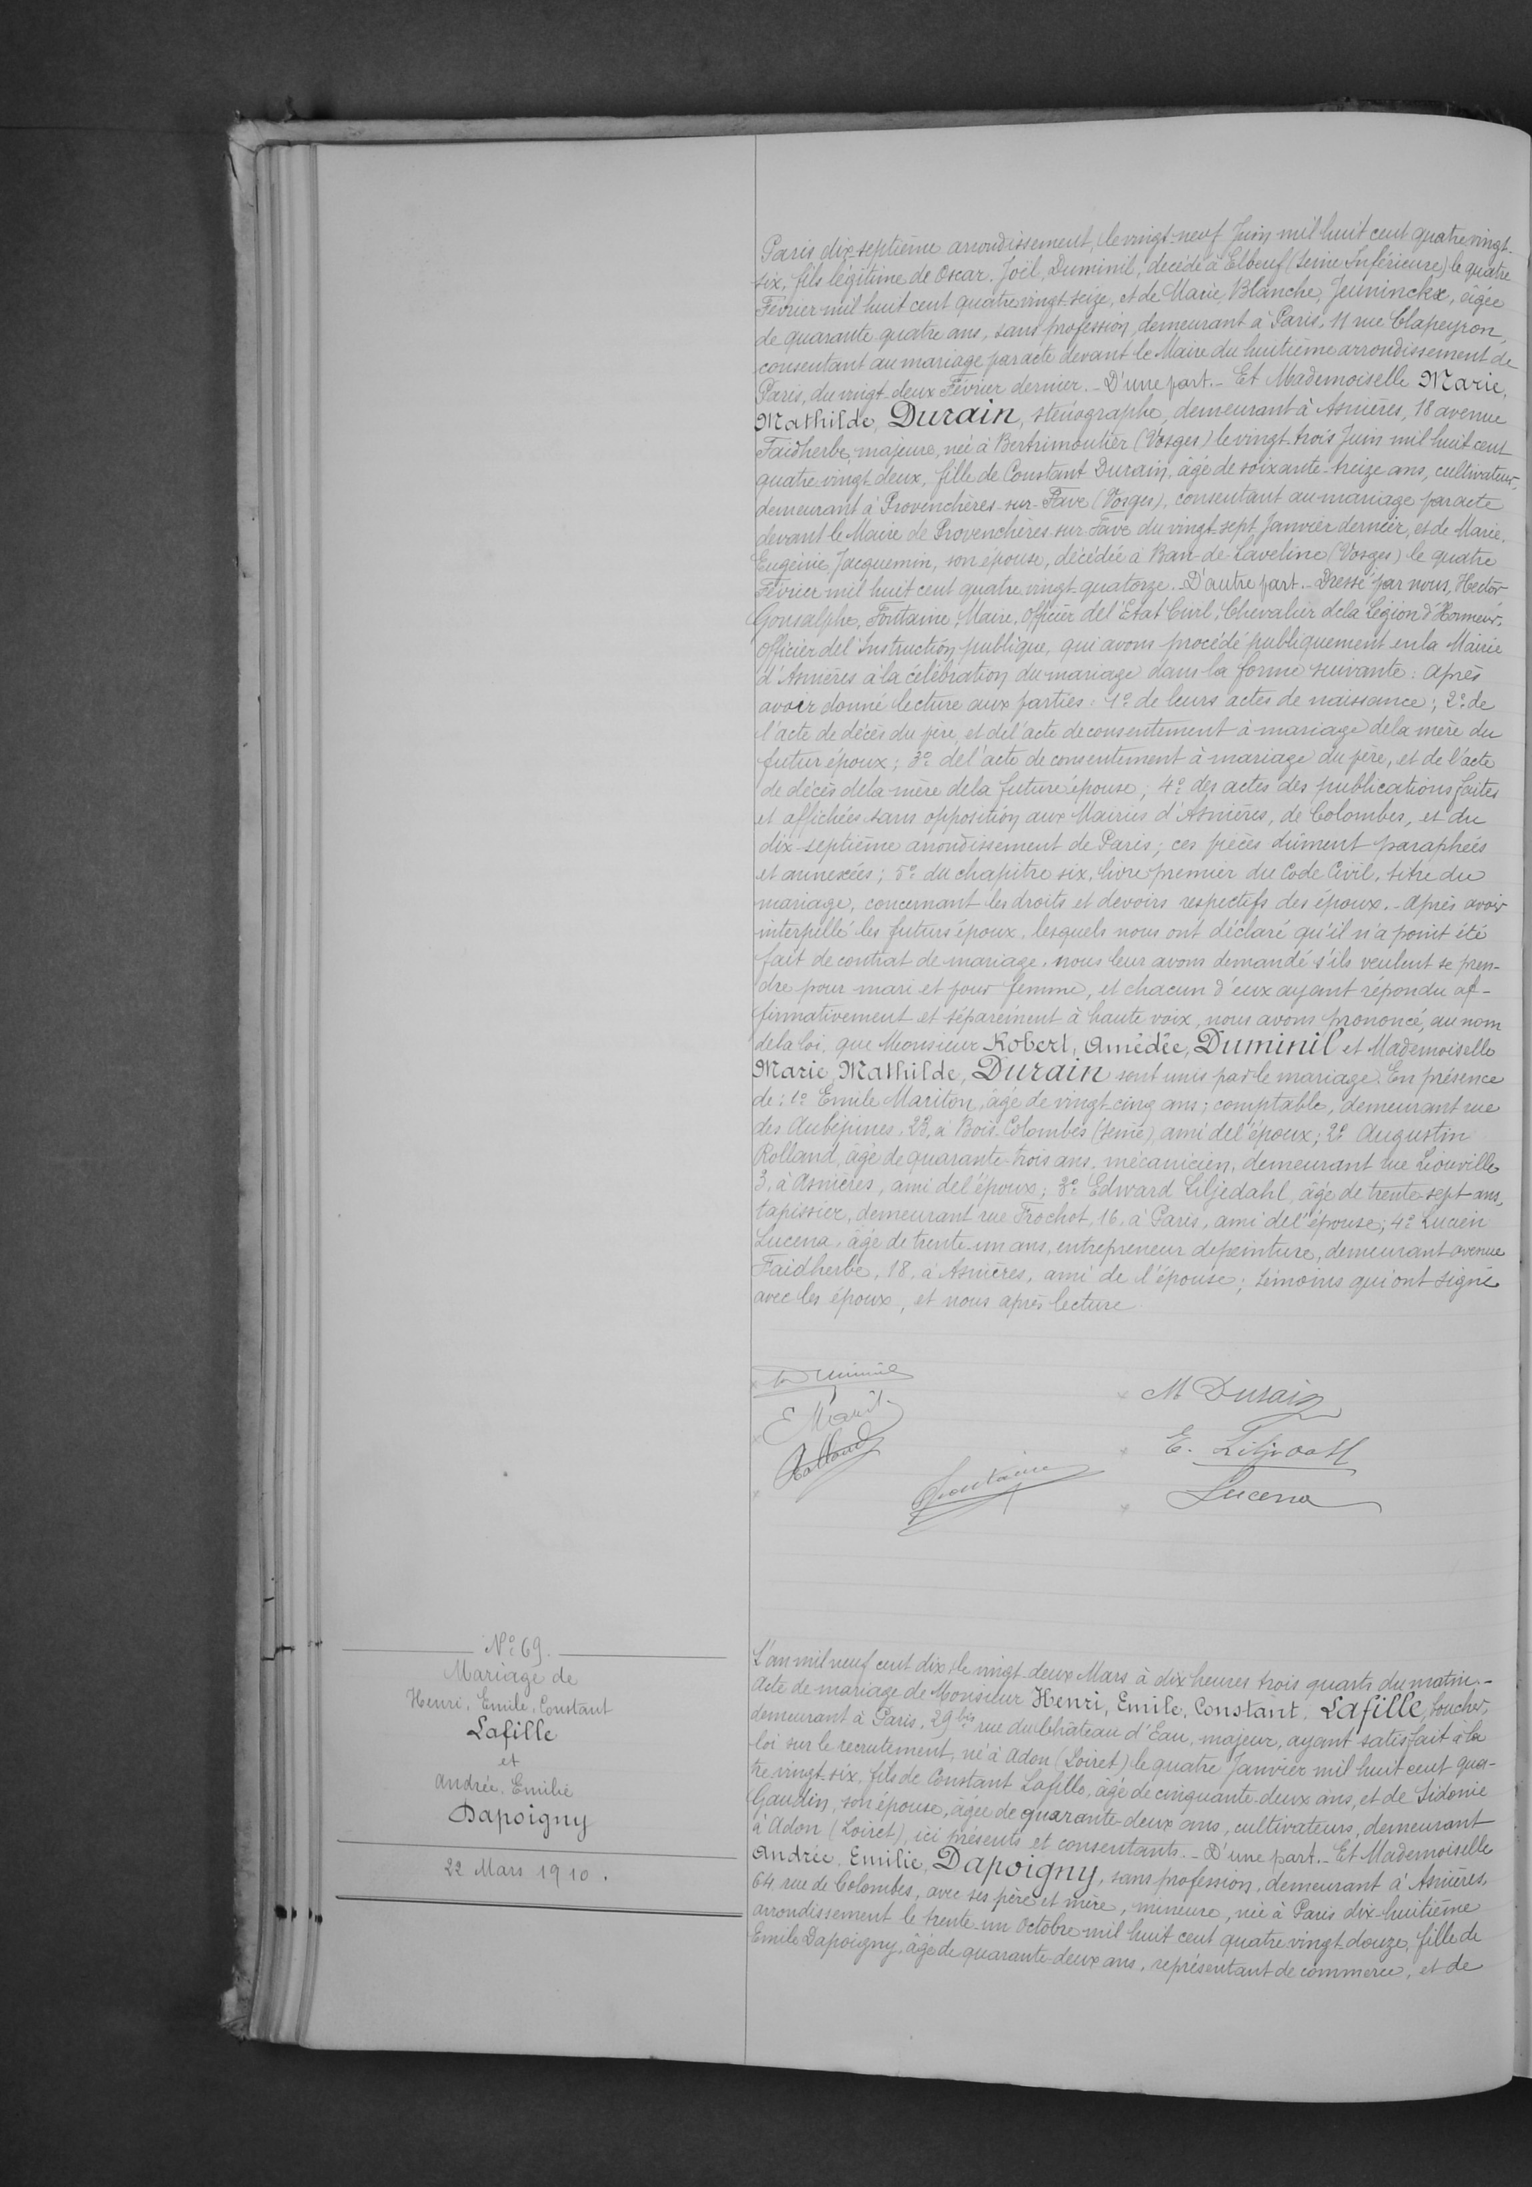Paris dix septième arrondissement, le vingt neuf Juin mil huit cent quatre vingt
six, fils légitime de Oscar. Joël Duminil, décédé à Elbeuf (Seine Inférieure) le quatre
Février mil huit cent quatre vingt seize et de Marie Blanche Jeuninckx, âgée
de quarante quatre ans, sans profession demeurant à Paris, 11 rue Clapeyron,
consentant au mariage par acte devant le Maire du huitième arrondissement de
Paris, du vingt-deux Février dernier.-D'une part .-Et Mademoiselle Marie,
Mathilde Durain, sténographe, demeurant à Asnières, 18 avenue
Faidherbe, majeurs, née à Bertrimoutier (Vosges) le vingt trois Juin mil huit cent
quatre vingt-deux, fille de Constant Durain, agé de soixante-treize ans, cultivateur
demeurant à Provenchères-sur-Fave (Vosges) consentant au mariage par acte
devant le Maire de Provenchères-sur-Fave du vingt-sept Janvier dernier et de Marie
Eugènie Jacquemin, son épouse, décédée, à Ban-de-Laveline (Vosges) le quatre
Février mil huit cent quatre vingt-quatorze. D'autre part.-Dressé par nous, Hector
Gonsalphe Fontaine, Maire, Officier de l'Etat Civil, Chevalier de la Légion d'Honneur
Officier de l'Instruction publique, qui avons procédé publiquement en la Mairie
d'Asnières à la célébration du mariage dans la forme suivante: après
avoir donné lecture aux parties: 1° de leurs actes de naissance; 2° de
l'acte de décès du père et de l'acte de consentement à mariage du mère du
futur époux; 3° del'acte de consentement à mariage du père, et de l'acte
de décés dela mère dela future épouse; 4° des actes de publications faites
et affichées sans opposition aux Mairies d'Asnières, de Colombes, et du
dix-septième arrondissement de Paris, ces pièces dûment paraphées
et annexées; 5° du chapitre six, livre premier du Code Civil, titre du
mariage, concernant les droits et devoirs respectifs des époux. -après avoir
interpellé les futurs époux, lesquels nous ont déclaré qu'il n'a point été
fait de contrat de mariage, nous leur avons demandé s'ils veulent se pren-
dre pour mari et pour femme, et chacun d'eux ayant répondu af-
firmativement et séparément à haute voix, nous avons prononcé, au nom
de la loi, que Monsieur Robert Amédé Duminil et Mademoiselle
Marie Mathilde Durain sont unis par le mariage. En présence
de: 1° Emile Mariton, agé de vingt-cinq ans; comptable, demeurant rue
des Aubépines, 23, à Bois-Colombes (Seine), ami del'époux; 2° Augustin
Rolland, âgé de quarante-trois ans. mécanicien, demeurant rue Léouville
3 à Asnières, ami de l'époux; 3° Edward Liljedahl, âgé de trente sept ans,
tapissier, demeurant rue Frochet, 16, à Paris, ami de l'épouse; 4° Lucien
Lucena, âgé de trente-un ans, entrepreneur de peinture, demeurant avenue
Faidherbe, 18, à Asnières, ami de l'épouse; témoins qui ont signé
avec les époux, et nous après lecture.
N°69
Mariage de
Henri Emile Constant
Lafille
et
Andrée Emilie
Dapoigny
22 Mars 1910
L'an mil neuf cent dix, le vingt-deux Mars à dix heures trois quarts du matin.-
acte de mariage de Monsieur Henrie, Emile, Constante, Lafille, boucher,
demeurant à Paris, 29 bis ru du Château d'Eau, majeur, ayant satisfait à la
loi sur le recrutement, né à adon (Loiret) le quatre Janvier mil huit cent qua-
tre vingt-six, fils de Constant Lafille, âgé de cinquante-deux ans, et de Sidonie
Gaudin, son épouse, âgée de quarante-deux ans, cultivateurs, demeurant
à Adon (Loiret), ici présents et consentants. D'une part. Et Mademoiselle
Andrée Emilie Dapoigny, sans profession, demeurant à Asnières,
64, rue de Colombes, avec ses père et mère, mineure, née à Paris dix-huitième
arrondissement le trente-un Octobre mil huit cent quatre vingt-douze, fille de
Emile Dapoigny, âgé de quarante-deux ans, représentant de commerce, et de
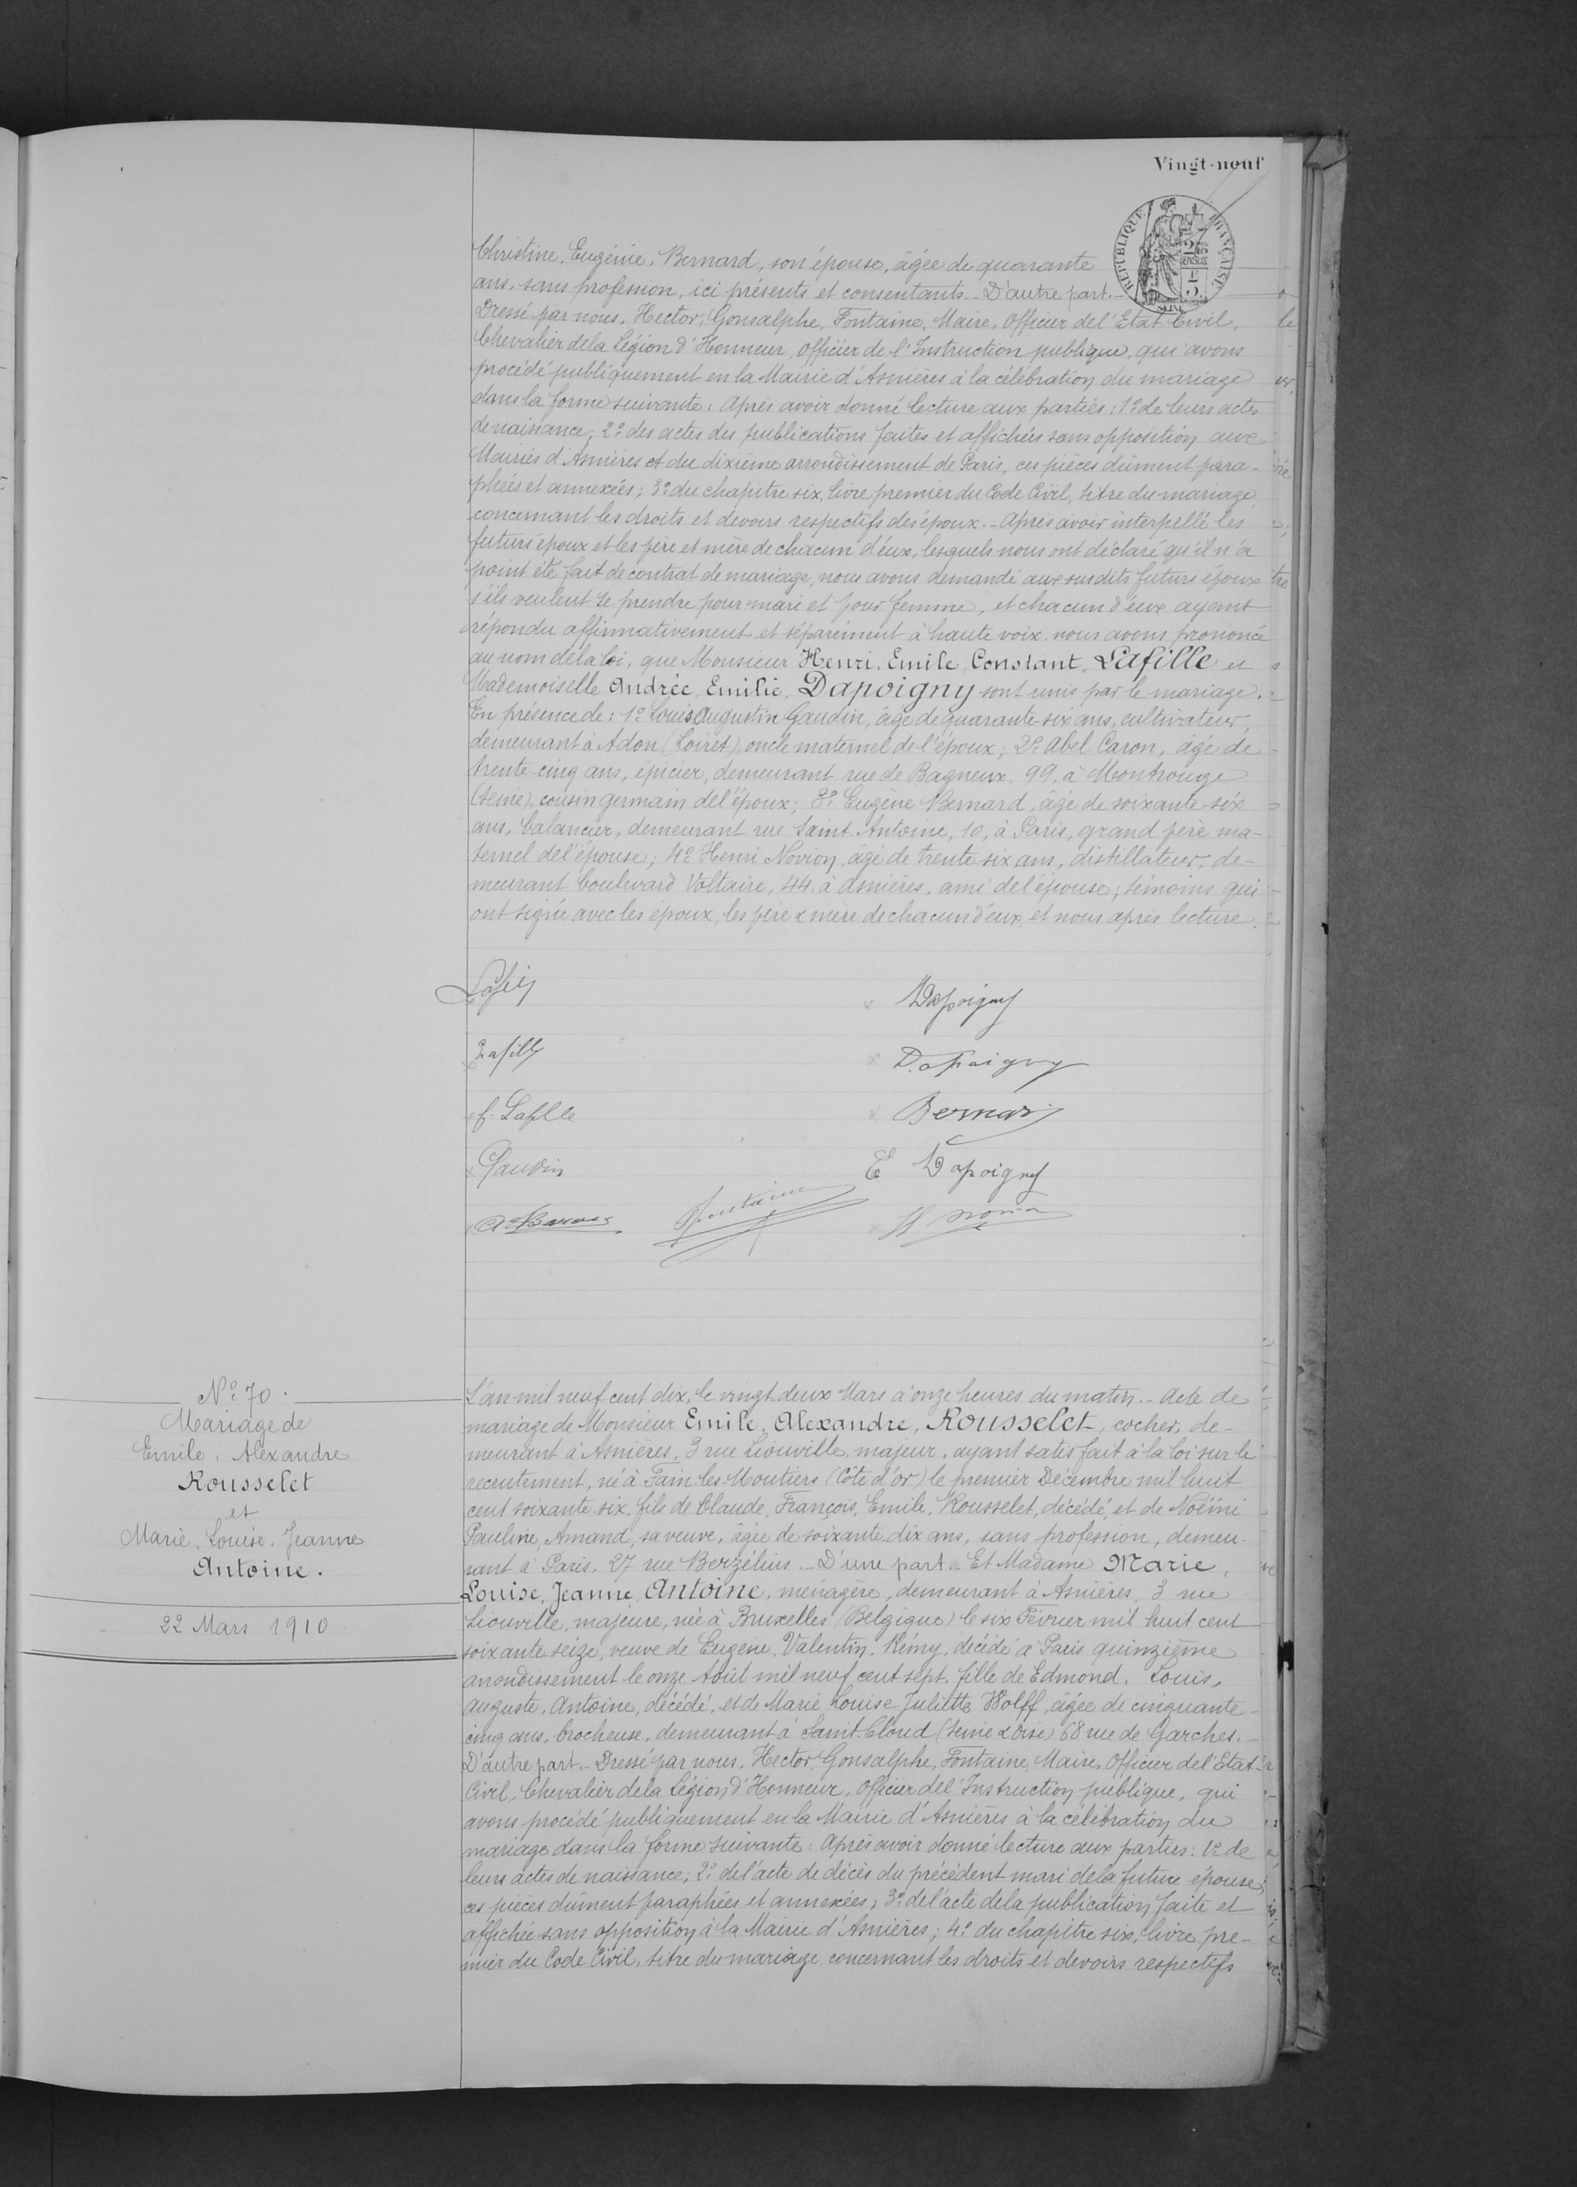Christine, Eugénie, Bernard, son épouse, âgée de quarante
ans, sans profession, ici présents et consentant. D'autre part.-
Dressé par nous. Hector, Gonsalphe, Fontaine, Maire, Officier de l'Etat Civil
Chevalier dela Légion d'Honneur, Officier de l'Instruction publique, qui avons
procédé publiquement en la Mairie d'Asnières à la célébration du mariage
dans la forme suivante: après avoir donné lecture aux parties: 1° de leurs actes
de naissance, 2° des actes des publications faites et affichées sans opposition aux
Mairies d'Asnières et du dixième arrondissement de Paris, ces pièces dûment para-
phées et annexées, 3° du chapitre six, livre premier du Code Civil, titre du mariage
concernant les droits et devoirs respectfis des époux.-Après avoir interpellé les
futurs époux et les père et mère de chacun d'eux, lesquels nous ont déclaré qu'il n'a
point été fait de contrat de mariage, nous avons demandé aux susdits futurs époux
s'ils veulent se prendre pour mari et pour femme, et chacun d'eux ayant
répondu affirmativement et séparément à haute voix, nous avons prononcé
au nom dela loi, que Monsieur Henri, Emile, Constant, Lafille et
Mademoiselle Andrée, Emilie Dapoigny, sont unie par le mariage.
En présence de: 1° Louis Augustin Gaudin, âgé de quarante-six ans, cultivateur,
demeurant à Adon (Loiret), oncle maternel de l'époux; 2° Abel Caron, âgé de
trente-cinq ans, épicier, demeurant rue de Bagneux, 99, à Montrouge
(Seine) cousin germain del'époux; 3° Eugène Bernard, âgé de soixante-six
ans, Balaneur, demeurant rue Saint-Antoine, 10, à Paris, grand père ma-
ternel del'épouse; 4° Henri Novion, âgé de trente-six ans, distillateur, de-
meurant boulevard Voltaire, 44,) Asnières, ami de l'épouse; témoins qui
ont signé avec les époux, les père et mère de chacun d'eux, et nous après lecture
n°70
Mariage de
Emile, Alexandre
Rousselet
et
Marie, Louise, Jeanne
Antoine
22 mars 1910
L'an mil neuf cent dix, le vingt-deux Mars à onze heures du matin.-Acte de
mariage de Monsieur Emile, Alexandre, Rousselet, cocher, de-
meurant à Asnières, 3 rue Liouville majeur, ayant satisfait) la loi sur le
recrutement, né à Fain-les-Moutiers (Côte-d'or) le premier décembre mil huit
cent soixante-six, fils de Claude, François, Emile, Rousselet, décédé et de Noëmi
Pauline Amand, sa veuve, âgée de soixante-dix ans, sans profession, demeu-
rant à Paris, 27 rue Berzéluis.-D'une part.-Et Madame Marie
Louise, Jeanne, Antoine, ménagère, demeurant à Asnières 3 rue
Liouville, majeure, né à Bruxelles (Belgique) le six février mil huit cent
soixante seize, veuve de Eugène, Valentin, Rémy, décédé à Paris quizième
arrondissement, le onze Aouût mil neuf cent sept, fille de Edmond, Louis,
Auguste, Antoine, décédé, et de Marie Louise Juliette Jolff, âgée de cinquante
cinq ans, brocheuse, demeurant à Saint-Cloud (Seine et Oise) 68 rue de Garches.
D'autre part.-Dressé par nous. Hectore Gonsalphe, Fontaine, Maire, Officier del'Etat
Civil, Chevalier de la Légion d'Honneur, Officier del'Instruction publique. qui
avons procédé publiquement en la Mairie d'Asnières à la célébration du
mariage dans la forme suivante: après avoir donné lecture aux partie: 1° de
leurs actes de naissance; 2° del'acte de décès du précédent mari dela future épouse
ces pièces dûment paraphées et annexées; 3° del'acte de la publication faite et
affichée sans opposition à la Mairie d'Asnières; 4° du chapitre six, livre pre-
mier du Code Civil, titre du mariage concernant les droits et devoirs respectifs